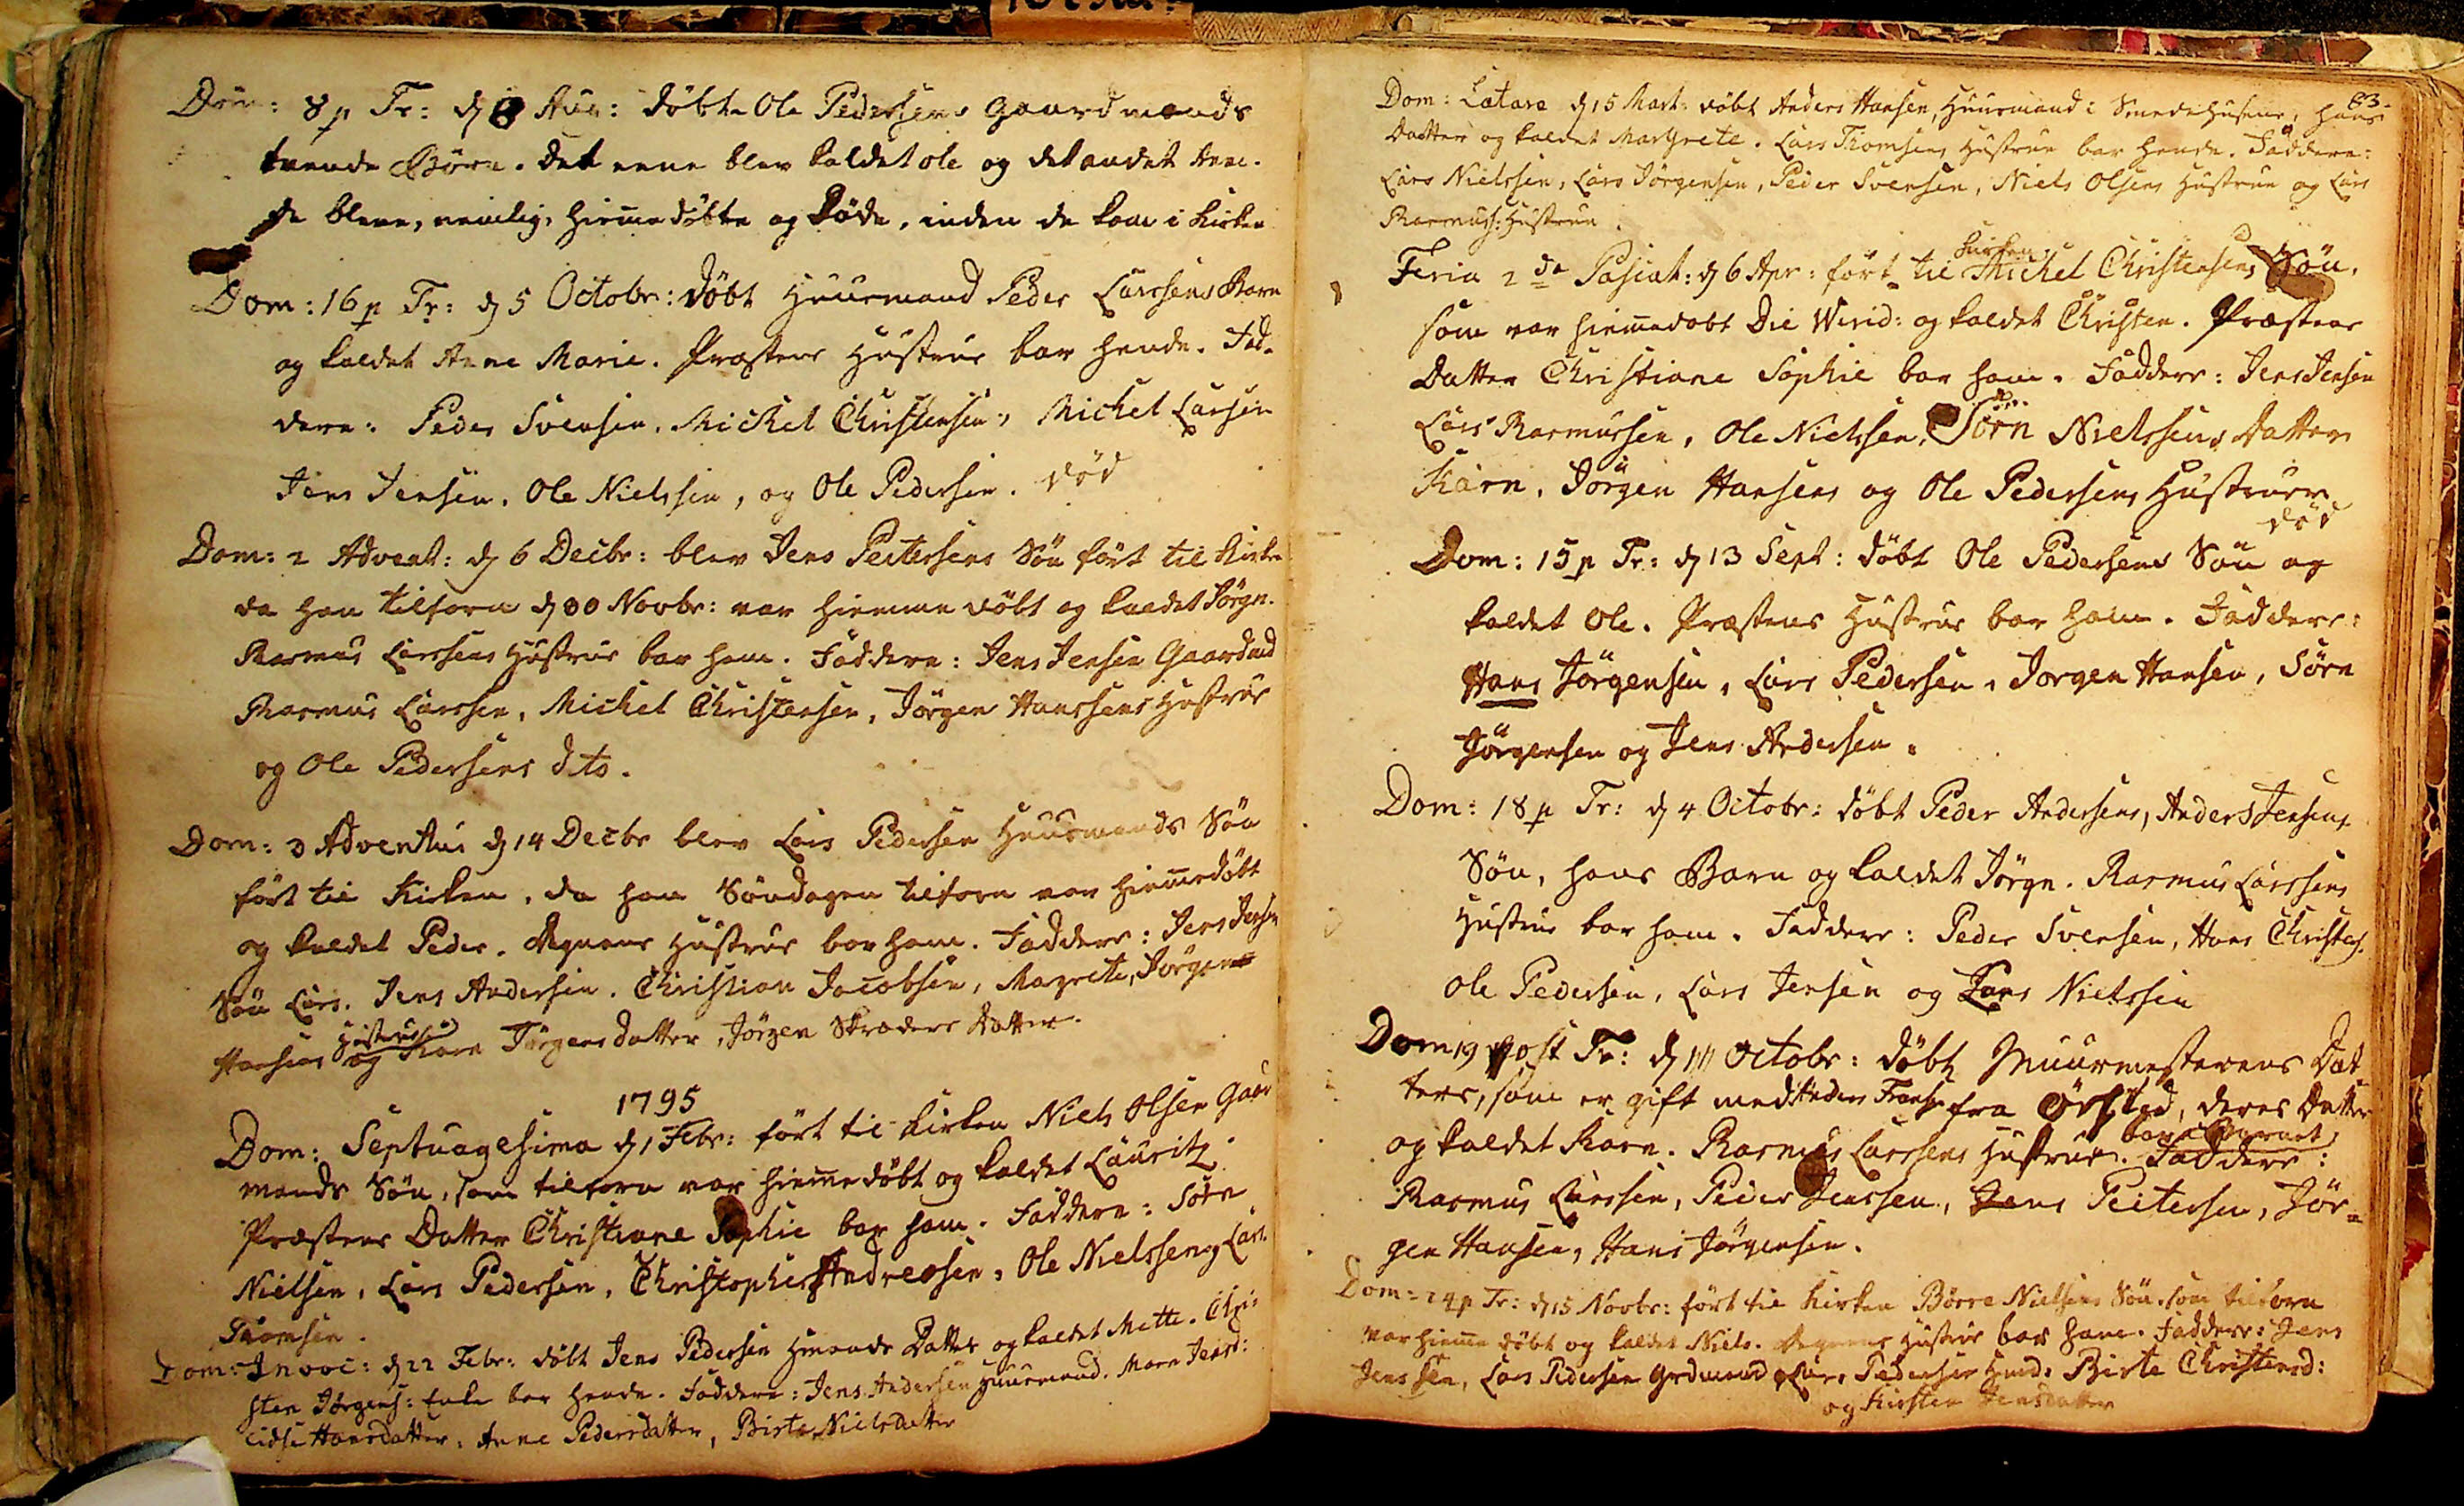Dom: 8 p Tr: d 8 Aug: døbte Ole Pedersens Gaardmands
tvende Børn. Det eene blev kaldet Ole og det andet Anne.
De bleve, nemlig, hiemmedøbte og Døde, inden de kom i Kirken.
Dom: 16 p Tr: d 5 Octobr: døbt Huusmand Peder Larssens Barn
og kaldet Anne Marie. Præstens Hustrue bar hende. Fad¬
dere: Peder Svensen, Michel Christensen, Michel Larsen
Jens Jensen, Ole Nielssen, og Ole Pedersen. død
Dom: 2 Advent: d 6 Decbr: blev Jens Peitersens Søn ført til Kirken
da han tilforn d 30 Novbr: var hiemmedøbt og kaldet Jørgn.
Rasmus Larssens Hustrue bar ham. Faddere: Jens Jensen Gaardmd
Rasmus Larssen, Michel Christensen, Jørgen Hanssens Hustrue 
og Ole Pedersens dito.
Dom: 3 Adventus d 14 Decbr: blev Lars Pedersen Huusmands Søn
ført til Kirken, da han Søndagen tilforn var hiemmedøbt
og kaldet Peder. Degnens Hustrue bar ham. Faddere: Jens Jensens
Søn Lars. Jens Andersen, Christian Jacobsen, Margrete, Jørgen
Hustrue
Hansens og Karn Jørgensdatter, Jørgen Skræders Datter.
1795
Dom: Septuagesima d 1 Febr: ført til Kirken Niels Olsen Gaard
mands Søn, som tilforn var hiemmedøbt og kaldet Lauritz
Præstens Datter Christiane Sophie bar ham. Faddere: Sørn
Nielsen, Lars Pedersen, Christopher Andreasen, Ole Nielssen og Lars
Thomsen.
Dom: Invoc: d 22 Febr: døbt Jens Pedersen Hmands Datter og kaldet Mette. Chri¬
sten Jørgens: Enke bar hende. Faddere: Jens Andersen Huusmand. Maren Jensd:
Cidse Hansdatter, Anne Pedersdatter, Birte Nielsdatter
83.
Dom: Lætare d 15 Mart: døbt Anders Hansen Huusmand i Smedehusene, hans
Datter og kaldet Margrete. Lars Thomsens Hustrue bar hende. Faddere:
Lars Nielssen,Lars Jørgensen, Peder Svensen, Niels Olsens Hustrue og Lars
Rasmuss: Hustrue.
Kirken
Feria 2da Pascat: d 6 Apr: ført til Michel Christensens Søn,
som var hiemmedøbt Die Wirid: og kaldet Christen. Præstens
Datter Christiane Sophie bar ham. Faddere: Jens Jensen
Lars Rasmussen, Ole Nielssen, Sørn Nielssens Datter
Karn, Jørgen Hansens og Ole Pedersens Hustruer
død
Dom: 15 p Tr: d 13 Sept: døbt Ole Pedersens Søn og
kaldet Ole. Præstens Hustrue bar ham. Faddere:
Hans Jørgensen, Lars Pedersen, Jørgen Hansen, Sørn
Jørgensen og Jens Andersen.
Dom: 18 p Tr: d 4 Octobr: døbt Peder Andersens, Anders Jensens
Søn, hans Barn og kaldet Jørgn. Rasmus Larssens
Hustrue bar ham. Faddere: Peder Svensen, Hans Christens:
Ole Pedersen, Lars Jensen og Lars Nielssen
Dom: 19 post Tr: d 11 Octobr: døbt Muurmesterens Dat¬
ter, som er gift med Anders Fransen fra Ørsted, deres Datter
bar Barnet
og kaldet Karn. Rasmus Larssens Hustrue. Faddere:
Rasmus Larssen, Peder Jenssen, Jens Peitersen, Jør¬
gen Hansen, Hans Jørgensen.
Dom: 24 p Tr: d 15 Novbr: ført til Kirken Børre Nielsens Søn, som tilforn
var hiemme døbt og kaldet Niels. Degnens Hustrue bar ham. Faddere: Jens
Jenssen, Lars Pedersen Grdmand, Lars Pedersen Hmd: Birte Christensd:
og Kirsten Jensdatter
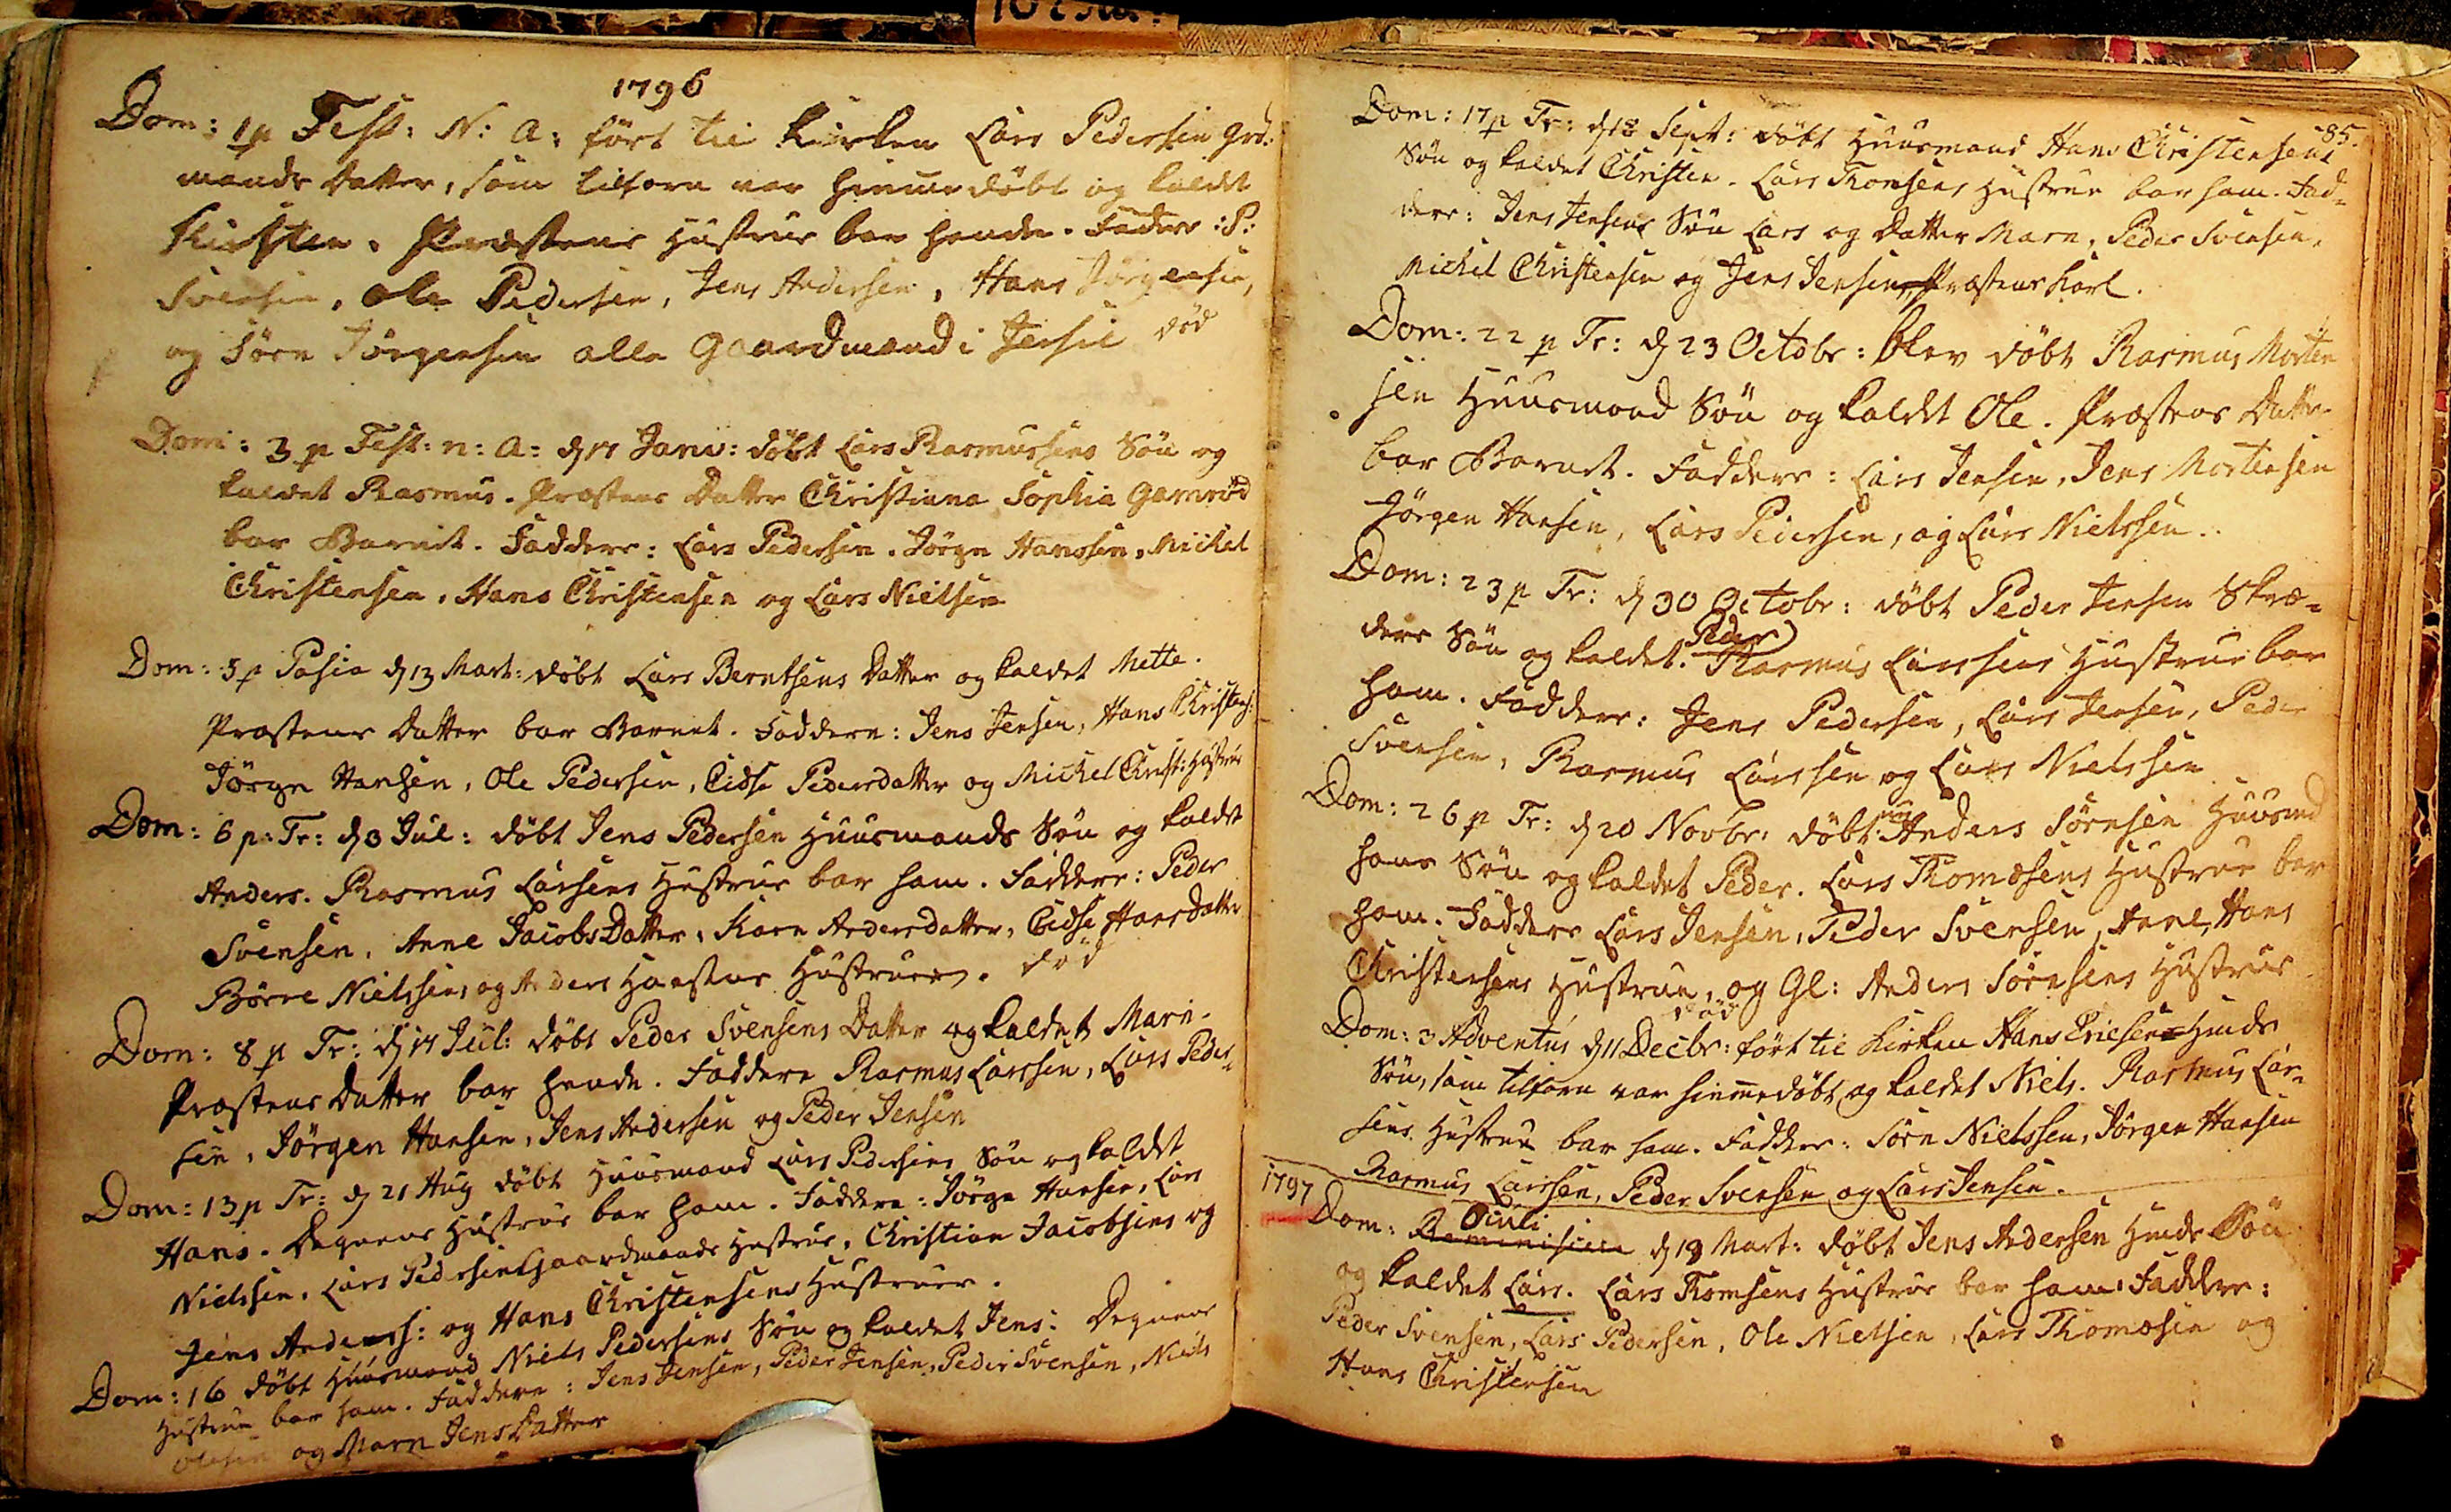1796
Dom: 1 p Fest: N: A: ført til Kirken Lars Pedersen Grd:
mands Datter, som tilforn var hiemmedøbt og kaldt
Kirsten. Præstens Hustrue bar hende. Fadere: P:
Svensen, Ole Pedersen, Jens Andersen, Hans Jørgensen,
og Sørn Jørgensen alle Gaardmænd i Jersie død
Dom: 3 p Fest: n: A: d 17 Janv: døbt Lars Rasmussens Søn og
kaldet Rasmus. Præstens Datter Christiane Sophie Gamrød
bar Barnet. Faddere: Lars Pedersen, Jørgn Hanssen, Michel
Christensen, Hans Christensen og Lars Nielsen
Dom: 5 p Pasca d 13 Mart: døbt Lars Berntsens Datter og kaldet Mette.
Præstens Datter bar Barnet. Faddere: Jens Jensen, Hans Christens:
Jørgn Hansen, Ole Pedersen, Sidse Pedersdatter og Michel Christ: Hustrue
Dom: 6 p Tr: d 3 Jul: døbt Jens Pedersen Huusmands Søn og kaldt
Anders. Rasmus Larsens Hustrue bar ham. Faddre: Peder
Svensen, Anne Jacobsdatter, Karn Andersdatter, Cidse Hansdatter
Børre Nielssens og Anders Hanstns Hustruer. død
Dom: 8 p Tr: d 17 Jul: døbt Peder Svensens Datter og kaldet Marn.
Præstens Datter bar hende. Faddere Rasmus Larssen, Lars Peder¬
sen, Jørgen Hansen, Jens Andersen og Peder Jensen
Dom: 13 p Tr: d 21 Aug døbt Huusmand Lars Pedersens Søn og kaldt
Hans. Degnens Hustrue bar ham. Faddere: Jørgn Hansen, Lars
Nielssen, Lars Pedersen Gaardmands Hustrue, Christian Jacobsens og
Jens Anderss: og Hans Christensens Hustruer.
Dom: 16 døbt Huusmand Niels Pedersens Søn og kaldet Jens: Degnens
Hustrue bar ham. Faddere: Jens Jensen, Peder Jensen, Peder Svensen, Niels
Olesen og Marn Jens Datter
85.
Dom: 17 p Tr: d 18 Sept: døbt Huusmand Hans Christensens
Søn og kaldet Christen. Lars Thomsens Hustrue bar ham. Fad¬
dere: Jens Jensens Søn Lars og Datter Marn. Peder Svensen,
Michel Christensen og Jens Jensen, Præstens Karl.
Dom: 22 p Tr: d 23 Octobr: blev døbt Rasmus Morten
sen Huusmand Søn og kaldt Ole. Præstens Datter
bar Barnet. Faddere: Lars Jensen, Jens Mortensen
Jørgen Hansen, Lars Pedersen, og Lars Nielssen.
Dom: 23 p Tr: d 30 Octbr: døbt Peder Jensen Skræ¬
Peder
ders Søn og kaldet. Rasmus Larssens Hustrue bar
ham. Faddere: Jens Pedersen, Lars Jensen, Peder
Svensen, Rasmus Larssen og Lars Nielssen.
ung
Dom: 26 p Tr: d 20 Novbr. døbt Anders Sørnsen Huusmd
hans Søn og kaldet Peder. Lars Thomæsens Hustrue bar
ham. Faddere Lars Jensen, Peder Svensen, Anne Hans
Christensens Hustrue, og Gl: Anders Sørnsens Hustrue
død
Dom: 3 Adventus d 11 Decbr: ført til Kirken Hans Ericsen Hmds
Søn, som tilforn var hiemmedøbt og kaldet Niels. Rasmus Lar¬
sens Hustrue bar ham. Faddere: Sørn Nielssen, Jørgen Hansen
Rasmus Larssen, Peder Svensen og Lars Jensen.
1797
Oculi
Dom: Reminiscere d 19 Mart: døbt Jens Andersen Hmds Søn
og kaldet Lars. Lars Thomsens Hustrue bar ham. Faddere:
Peder Svensen, Lars Pedersen, Ole Nielsen, Lars Thomæsen og
Hans Christensen